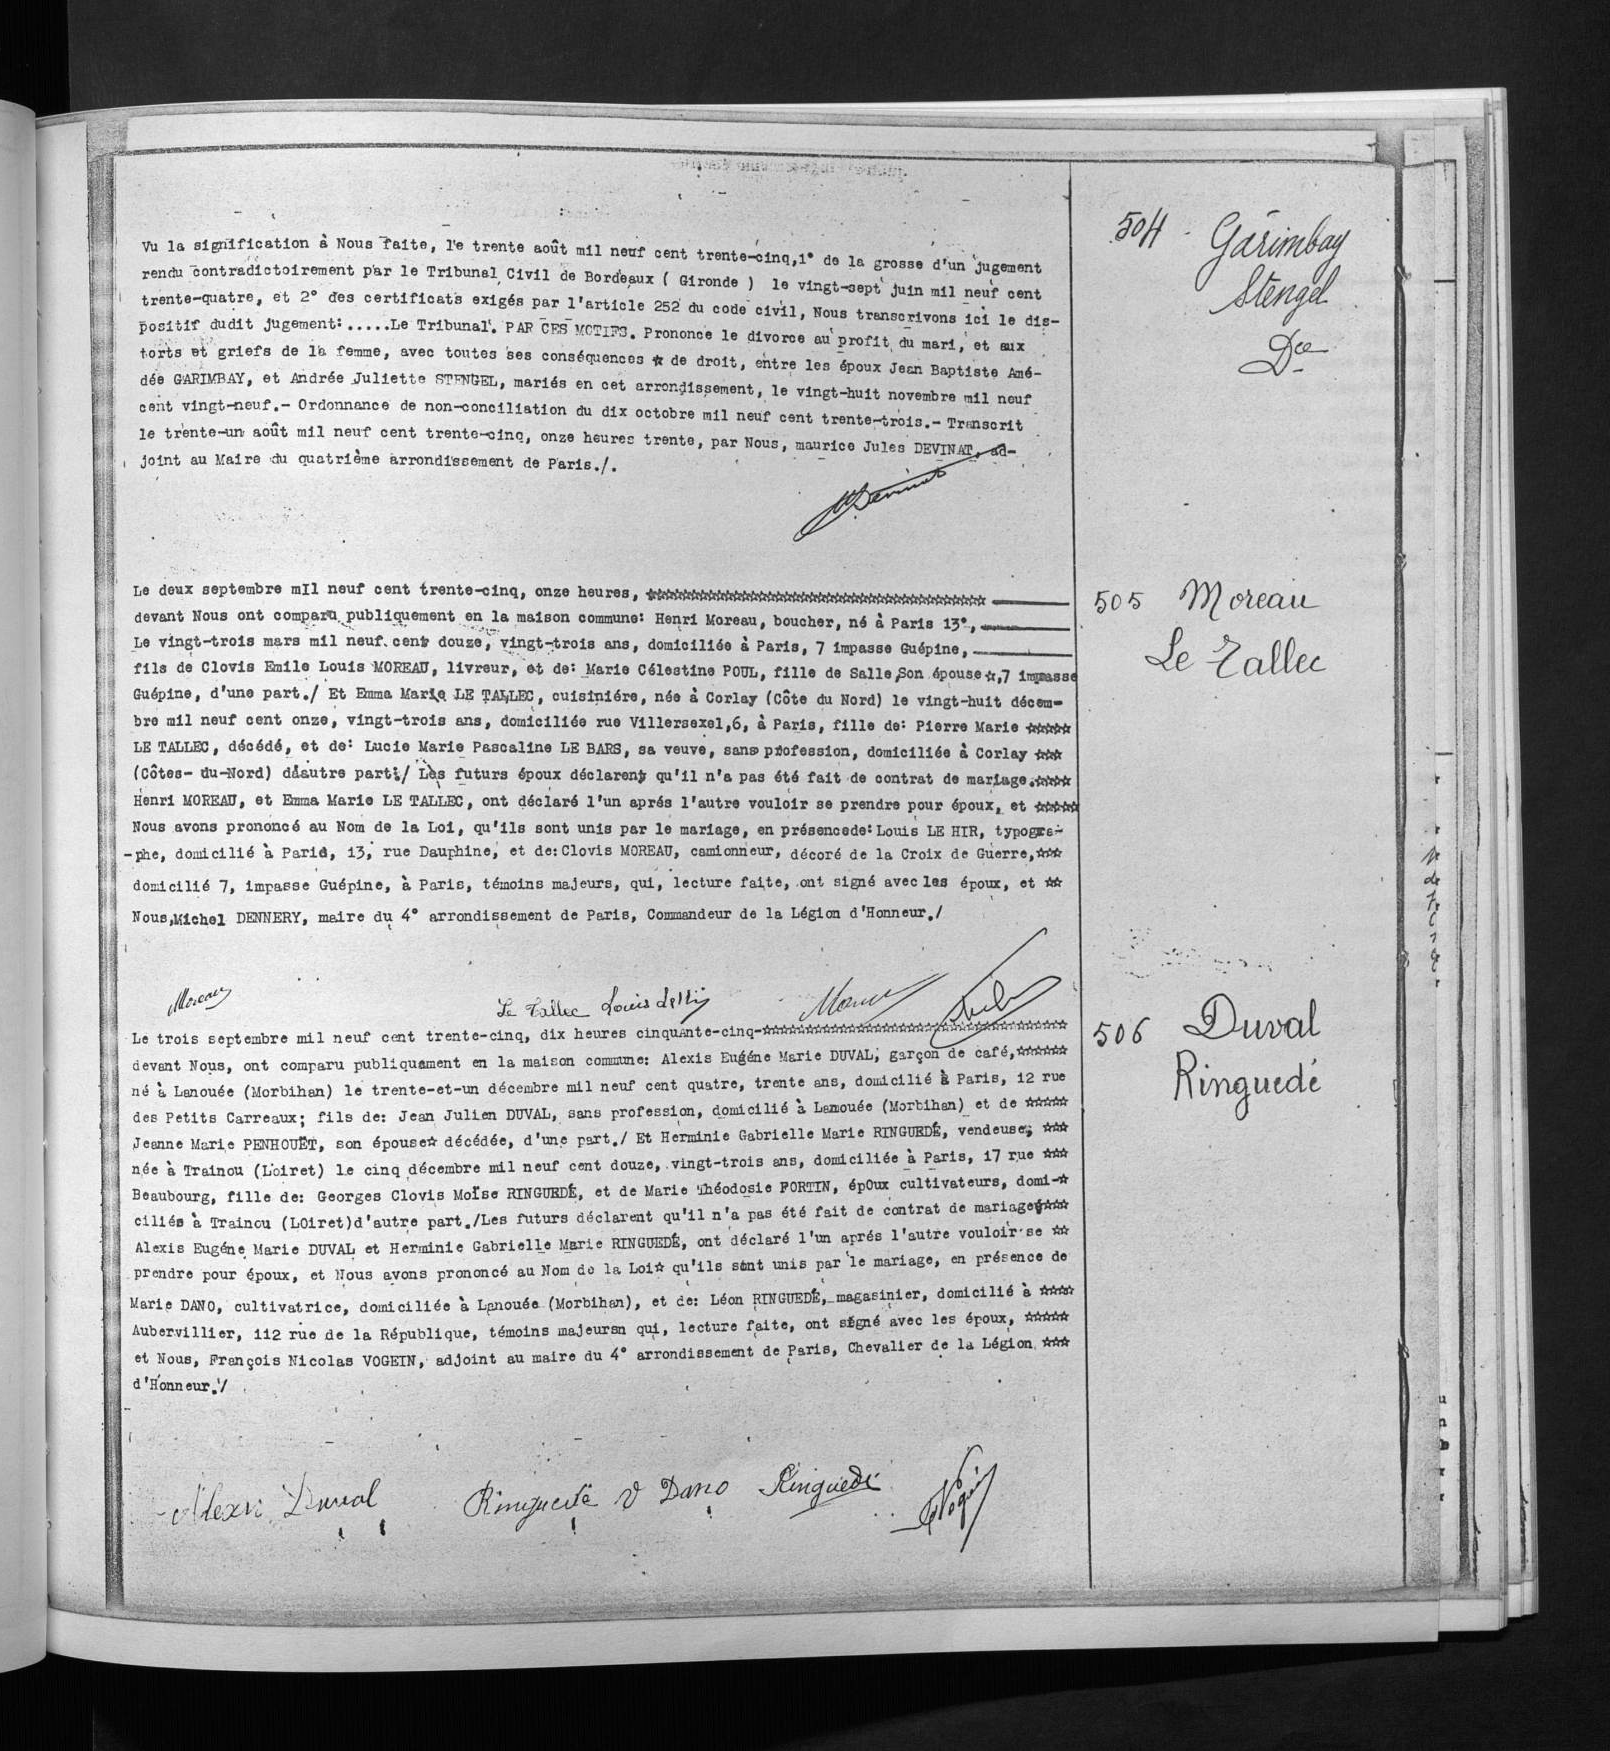Vu la signification à Nous faite, le trente août mil neuf cent trente-cinq, 1° de la grosse d'un jugement
rendu contradictoirement par le Tribunal Civil de Bordeaux (Gironde) le vingt-sept juin mil neuf cent
trente-quatre, et 2° des certificats exigés par l'article 252 du code civil, Nous transcrions ici le dis-
positif dudit jugement:..... Le Tribunal. PAR CES MOTIFS. Prononce le divorce au profit du mari, et aux
torts et griefs de la femme, avec toutes ses conséquences * de droit, entre les époux Jean Baptiste Amé-
dée GARIMBAY, et Andrée Juliette STENGEL, mariés en cet arrondissement, le vingt-huit novembre mil neuf
cent vingt-neuf .-Ordonnance de non-conciliation du di octobre mil neuf cent trente-trois .-Transcrit
le trente-un août mil neuf cent trente-cinq, onze heures trente, par Nous, maurice Jules DEVINAT, ad-
joint au Maire du quatrième arrondissement de Paris ./.
Le deux septembre mil neuf cent trente-cinq, onze heures, ****-
devant Nous ont comparu publiquement en la maison commune: Henri Moreau, boucher, né à Paris 13°, -
Le vingts-trois mars mil neuf cent douze, vingt-trois ans, domiciliée à Paris, 7 impasse Guépine, -
fils de Clovis Emile Louis MOREAU, livreur, et de: Marie Célestine POUL, fille de Salle, Son épouse *, 7 impasse
Guépine, d'une part ./ Et Emma Marie LE TALLEC, cuisinière, née à Corlay (Côte du Nord) le vingt-huit décem-
bre mil neuf cent onze, vingt-trois ans, domiciliée rue Villersexel, 6, à Paris, fille de: Pierre Marie ****
LE TALLEC, décédé, et de: Lucie Marie Pascaline LE BARS, sa veuve, sans profession, domiciliée à Corlay ***
(Côtes-du-Nord) daautre part:/ Les futurs époux déclarent qu'il n'a pas été fait de contrat de mariage .****
Henri MOREAU, et Emma Marie LE TALLEC, ont déclaré l'un après l'autre vouloir se prendre pour époux, et ***
Nous avons prononcé au Nom de la Loi, qu'ils sont unis par le mariage, en présence de: Louis LE HIR, typogra-
-phe, domicilié à Paris, 13, rue Dauphine, et de: Clovis MOREAU, camionneur, décoré de la Croix de Guerre, ***
domicilié 7, impasse Guépine, à Paris, témoins majeurs, qui, lecture faite, ont signé avec les époux, et **
Nous, Michel DENNERY, maire du 4° arrondissement de Paris, Commandeur de la Légion d'Honneur ./
Le trois septembre mil neuf cent trente-cinq, dix heures cinquante-cinq -****
devant Nous, ont comparu publiquement en la maison commune: Alexis Eugéne Marie DUVAL; garçon de café ,***
né à Lanouée (Morbihan) le trente-et-un décembre mil neuf cent quatre, trente ans, domicilié à Paris, 12 rue
des Petits Carreaux; fils de: Jean Julien DUVAL, sans profession, domicilié à Lamouée (Morbihan) et de ****
Jeanne Marie PENHOUET, son épouse * décédée, d'une part./ Et Herminie Gabrielle Marie RINGUEDE, vendeuse, ***
née à Trainou (Loiret) le cinq décembre mil neuf cent douze, vingt-trois ans, domiciliée à Paris, 17 rue ***
Beaubourg, fille de: Georges Clovis Moïse RINGUARDE, et de Marie Théodosie FORTIN, ép0ux cultivateurs, domi -*
ciliés à Trainou (Loiret) d'autre part ./ Les futurs déclarent qu'il n'a pas été fait de contrat de mariage ****
Alexis Eugène Marie DUVAL et Herminie Gabrielle Marie RINGUEDE, ont déclaré l'un après l'autre vouloir se **
prendre pour époux, et Nous avons prononcé au Nom de la Loi * qu'ils sont unis par le mariage, en présence de
Marie DANO, cultivatrice, domiciliée à Lanouée (Morbihan), et de Léon RINGUEDE, magasinier, domicilié à ***
Aubervillier, 112 rue de la République, témoins majeuran qui, lecture faite, ont signé avec les époux, ****
et Nous, François Nicolas VOGEIN, adjoint au maire du 4° arrondissement de Paris, Chevalier de la Légion ***
d'Honneur ./